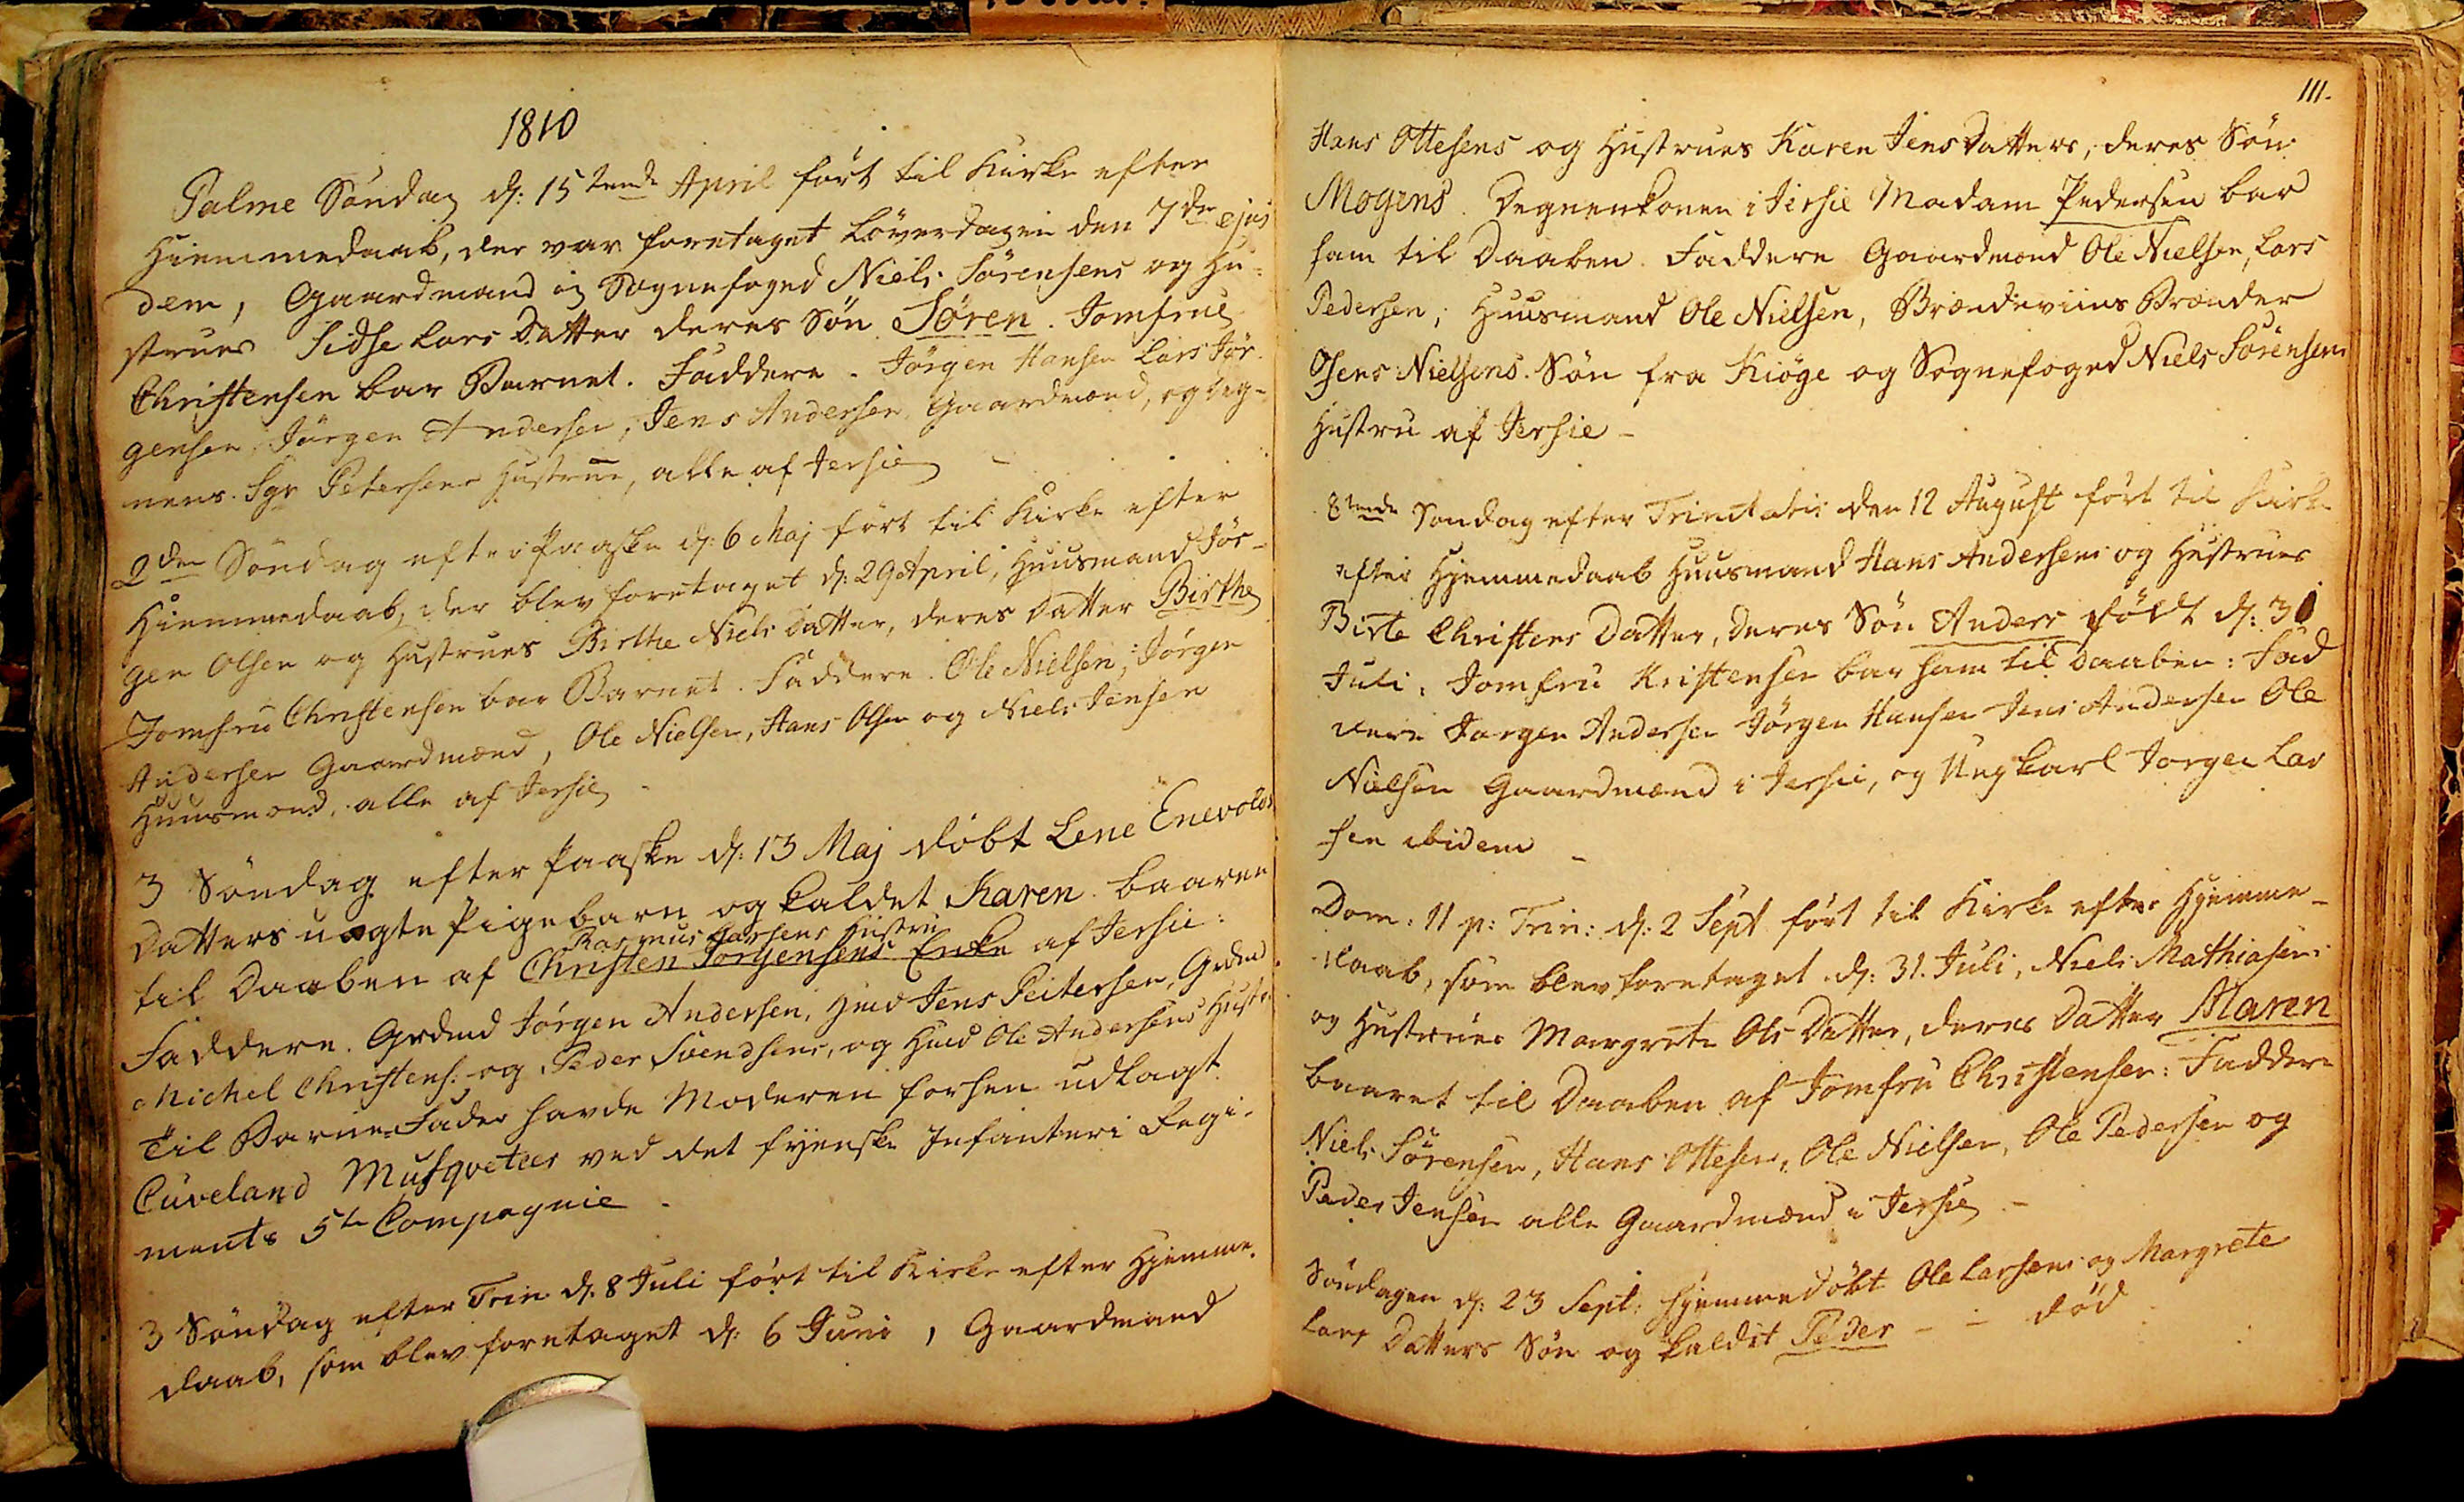1810
Palme Søndag d: 15tende April ført til Kirke efter
Hiemmedaab, der var foretaget Løverdagen den 7de ejus
dem, Gaardmand og Sognefoged Niels Sørensens og Hu¬
strues Sidse Lars Datter deres Søn Søren. Jomfrue
Christensen bar Barnet. Faddere. Jørgen Hansen Lars Jør
gensen, Jørgen Andersen, Jens Andersen, Gaardmænd, og Deg¬
nens. Sgr Petersens Hustrue, alle af Jersie 
2den Søndag efter Paaske d: 6 Maj ført til Kirke efter
Hiemmedaab, der blev foretaget d: 29 April, Huusmand Jør¬
gen Olsen og hustrues Birthe Niels Datter, deres Datter Birthe 
Jomfru Christensen bar Barnet. Faddere. Ole Nielsen, Jørgen
Andersen Gaardmænd, Ole Nielsen, Hans Olsen og Niels Jensen
Huusmænd, alle af Jersie.
3 Søndag efter Paaske d. 13 Maj døbt Lene Enevolds
Datters uægte Pigebarn og kaldet Karen. Baaren
Rasmus Larsens Hustru
til Daaben af Christen Jørgensens Enke af Jersie:
Faddere. Grdmd Jørgen Andersen, Hmd Jens Peitersen, Grdnd
Michel Christens: og Peder Svendsens, og Hmd Ole Andersens Hustr:
Til Barne-Fader havde Moderen forhen udlagt
Cuveland Musqveteer ved det fynske Infanteri Regi¬
ments 5te Compagnie.
3 Søndag efter Trin. d: 8 Juli ført til Kirke efter Hjemme
daab, som blev foretaget d: 6 Juni, Gaardmand
111.
Hans Ottesens og Hustrues Karen Jens Datters, deres Søn
Mogens. Degnenkonen i Jersie Madam Pedersen bar
ham til Daaben. Faddere Gaardmænd Ole Nielsen, Lars
Pedersen; Huusmand Ole Nielsen, Brændeviins Brænder
Jens Nielsens Søn fra Kiøge og Sognefoged Niels Sørensens
Hustru af Jersie.
8tende Søndag efter Trinitatis den 12 August ført til kirke
efter Hjemmedaab Huusmand Hans Andersens og Hustrues
Birte Christens Datter, Deres Søn Anders født d: 31
Juli: Jomfru Kristensen bar ham til Daaben: Fad
dere Jorgen Andersen Jørgen Hansen Jens Andersen Ole
Nielsen Gaarmænd i Jersie, og Ungkarl Jorgen Lar
sen ibidem
Dom: 11 p: Trin: d: 2 Sept. ført til Kirke efter Hjemme¬
daab, som blev foretaget d: 31. Juli, Niels Mathiasens
og Hustrues Margrete Ols Datter, deres Datter Maren 
baaret til Daaben af Jomfru Christensen: Faddere
Niels Sørensen, Hans Ottesen, Ole Nielsen, Ole Pedersen og
Peder Jensen alle Gaardmænd i Jersie. -
Søndagen d: 23 Sept: Hjemmedøbt Ole Larsens og Margrete
Lars Datters Søn og kaldet Peder
død